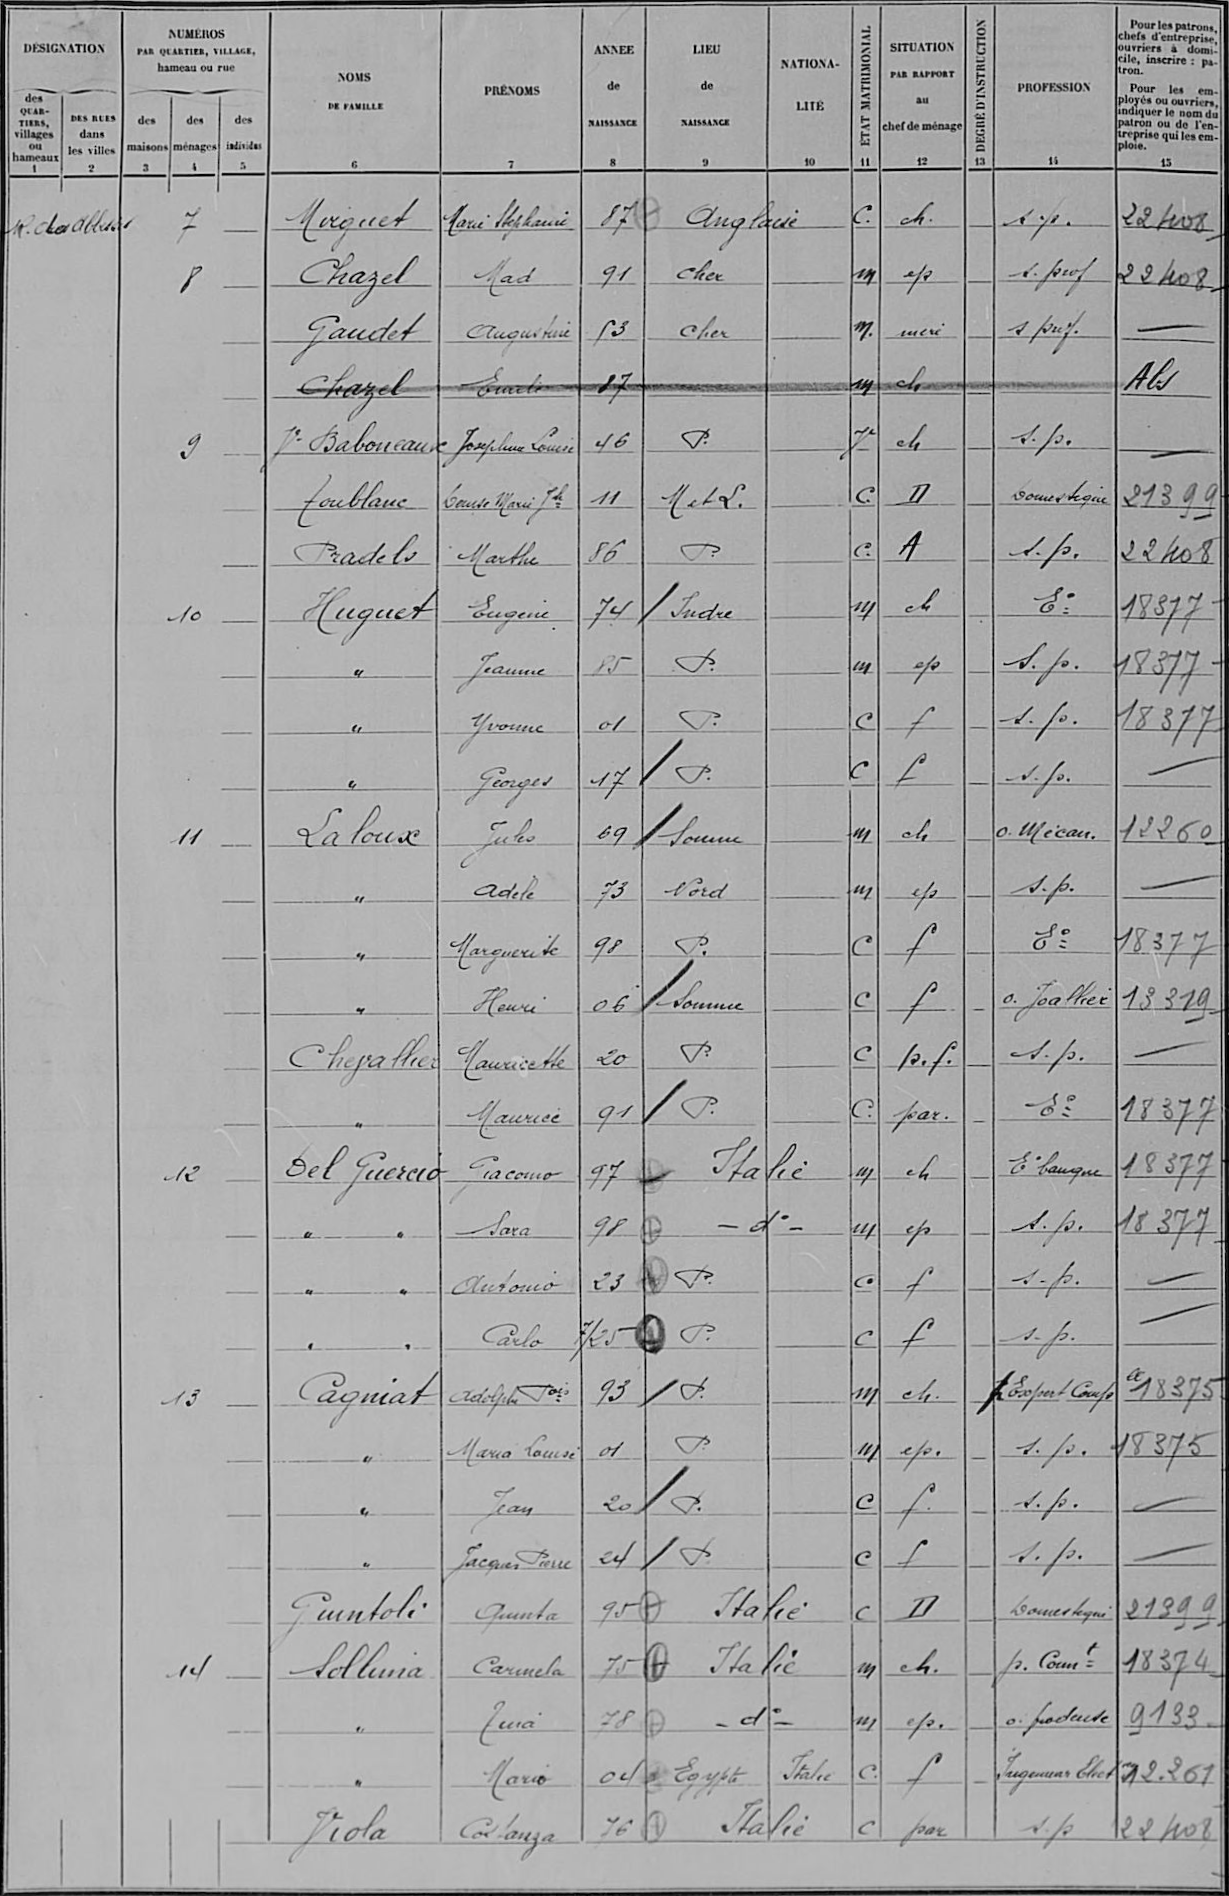Mirguet/Marie Stéphanie/87/Anglaise/¤/C /ch /¤/s p /22408
Chazel/Mad/91/cher/¤/m/ep/¤/s prof/22408
Gaudet/Augustine/53/cher/¤/m /mère/¤/s prof /¤
Chazel/Emile/87/¤/¤/m/ch/¤/¤/Abs
V Baboueaux/Joséphine Louise/46/P /¤/V/ch/¤/s p /¤
Toublanc/Louise Marie Jh/11/M et L /¤/C/D/¤/domestique/21399
Pradelo/Marthe/86/P /¤/C /A/¤/s p /22408
Huguet/Eugenie/74/Indre/¤/m/ch/¤/E°/18377
"/Jeanne/85/P /¤/m/ep /¤/s p /18377
"/Yvonne/01/P /¤/C/f/¤/s p /18377
"/Georges/17/P /¤/C/f/¤/s p /¤
Laloux/Jules/69/Somme/¤/m/ch/¤/o mécan /12260
"/Adèle/73/Nord/¤/m/ep/¤/s p /¤
¤/marguerite/98/P /¤/C/f/¤/E°/18377
¤/Henri/06/Somme/¤/C/f/¤/o joallier/13319
Chevallier/Mauricette/20/P/¤/C/p f /¤/s p /¤
¤/Maurice/91/P /¤/C /par /¤/E°/18377
Del Guercio/Giacomo/97/Italie/¤/m/ch/¤/E banque/18377
" "/Sara/98/d°/¤/m/ep/¤/s p /18377
" "/Antonio/23/P /¤/C/f/¤/s p /¤
" "/Carlo/7 25/P /¤/C/f/¤/s p /¤
Cagniat/Adolphe Pois/93/P /¤/m/ch /¤/Expert comp/18375
"/Maria Louise/01/P /¤/m/ep /¤/s p /18375
"/Jean/20/P /¤/C/f /¤/s p /¤
"/Jacques Pierre/24/P /¤/C/f/¤/s p /¤
Guintoli/Quinta/95/Italie/¤/C/D/¤/Domestique/21399
Sollunia/Carmela/75/Italie/¤/m/ch /¤/p Commt/18374
¤/Zuia/78/d°/¤/m/ep /¤/o brodeuse/9133
¤/Mario/04/Egypte/Italie/C/f/¤/Ingenieur chef/12 261
Viola/Costanza/76/Italie/¤/C/par/¤/s p /22408
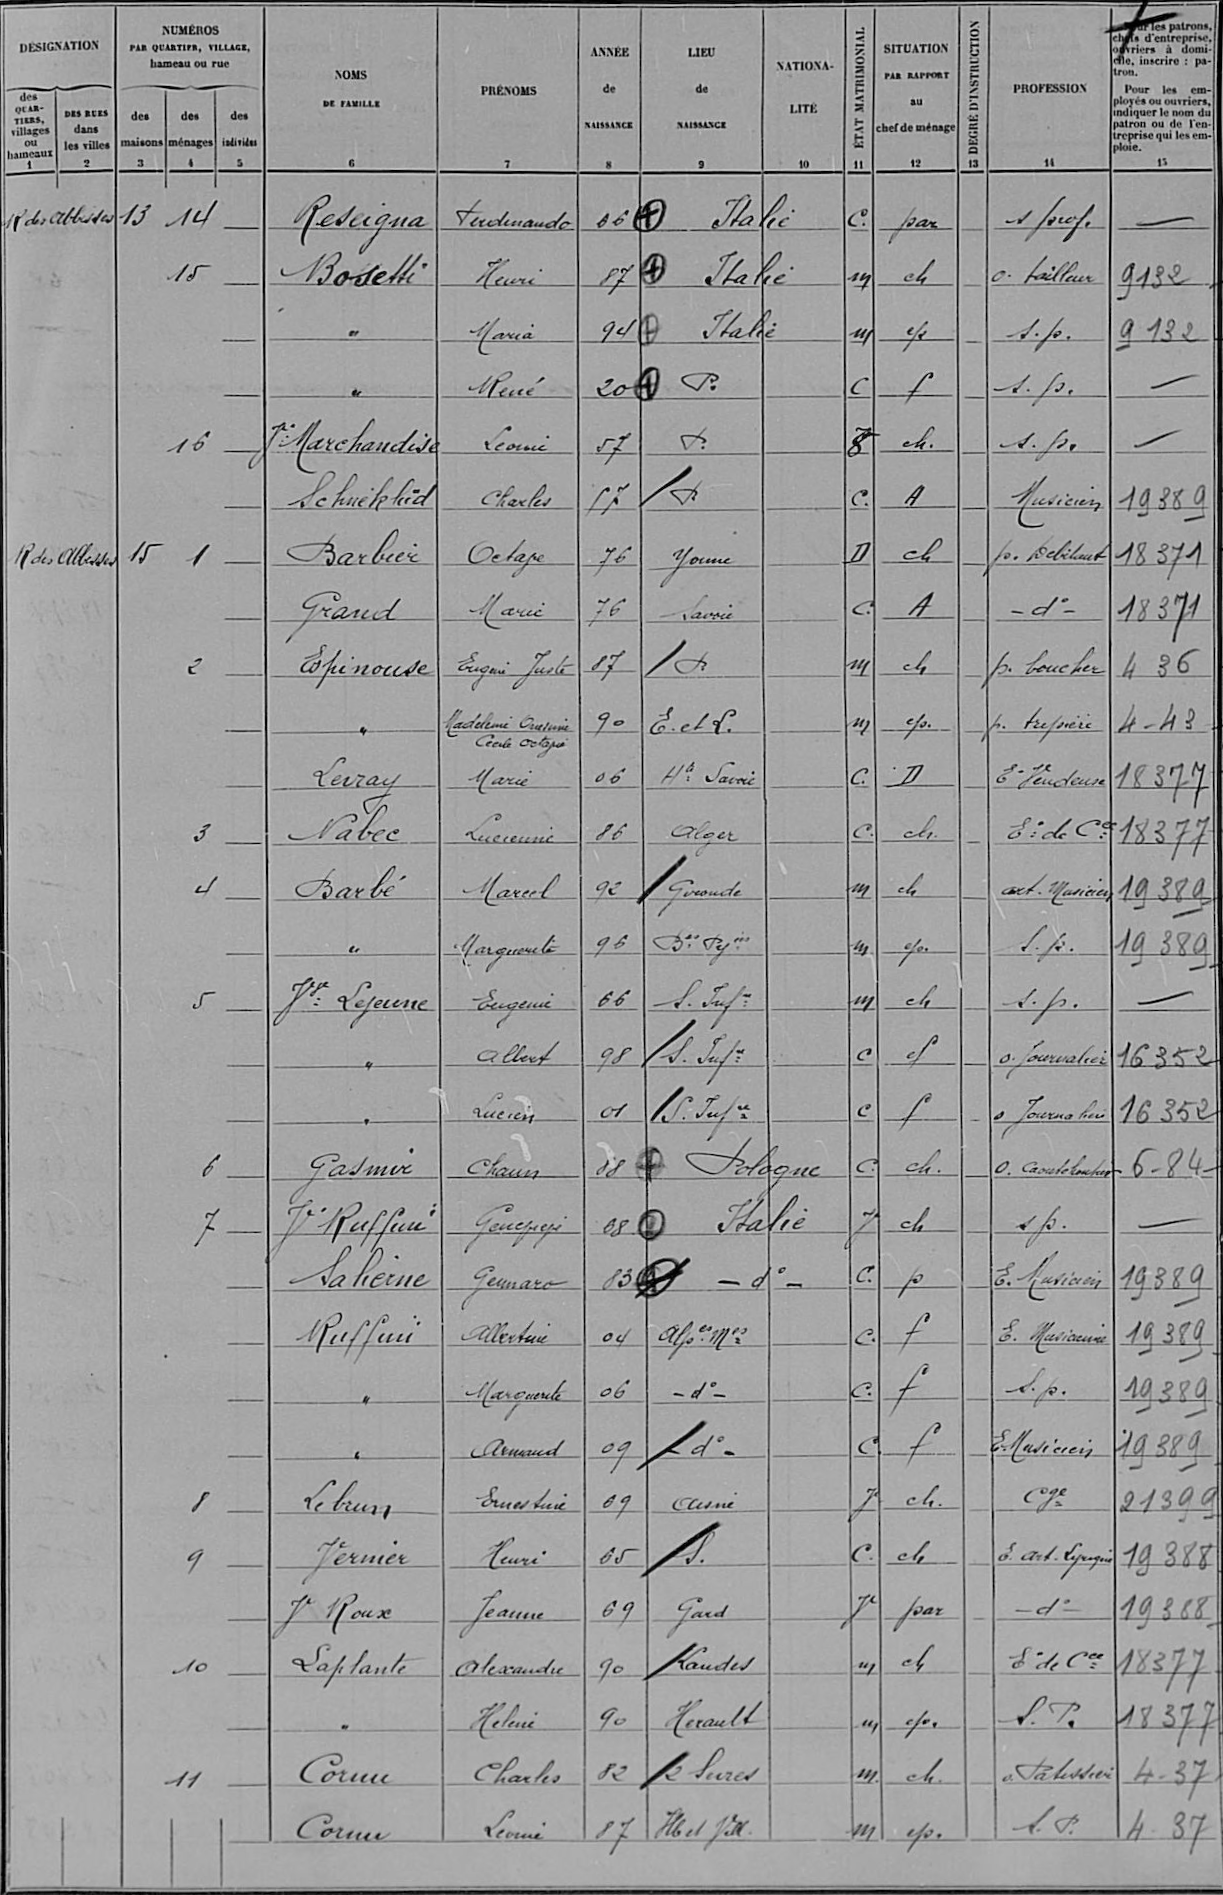Reseigna/Ferdinando/06/Italie/¤/C /par/¤/s prof /¤
Bosetti/Henri/87/Italie/¤/m/ch/¤/o tailleur/9132
¤/maria/94/Italie/¤/m/ep/¤/s p /9132
¤/René/20/P /¤/C/f/¤/s p /¤
V Marchandise/Leonie/57/P /¤/V/ch /¤/s p /¤
Schneklüd/Charles/57/P/¤/C /A/¤/Musicien/19389
Barbier/Octave/76/Yonne/¤/D/ch/¤/p Debitant/18371
Grand/Marie/76/Savoie/¤/C /A/¤/d°/18371
Espinouse/Eugénie Juste/87/P/¤/m/ch/¤/p boucher/436
¤/Madeleine Oresine !Cecile Octavie/90/E et L /¤/m/ep /¤/p fripière/4 43
Levray/Marie/06/Hte Savoie/¤/C /D/¤/E Vendeuse/18377
Nabec/Lucienne/86/Alger/¤/C /ch /¤/E de Cce/18377
Barbé/Marcel/92/Gironde/¤/m/ch/¤/art Musicien/19389
¤/Marguerite/96/Bes Pées/¤/m/ep /¤/s p /19389
Veuve Lejeune/Eugénie/66/S Infre/¤/m/ch/¤/s p /¤
¤/albert/98/S Infre/¤/c/f/¤/o journalier/16352
¤/Lucien/01/S Infre/¤/c/f/¤/o journalier/16352
Gasmir/Chaun/88/Pologne/¤/C /ch /¤/O Caoutchouteur/6 84
V Ruffini/Genepiepi/68/Italie/¤/V/ch/¤/sp /¤
Salierne/Gennaro/83/d°/¤/C /p/¤/E Musicien/19389
Ruffini/Albertine/04/Alpes Mes/¤/C/f/¤/E Musicienne/19389
¤/Marguerite/06/d°/¤/C /f/¤/s p /19389
¤/Armand/09/d°/¤/C /f/¤/E Musicien/19389
Lebrun/Ernestine/09/Aisne/¤/V/ch /¤/Cge/21399
Vernier/Henri/05/S/¤/C /ch/¤/E art Le progrès/19388
V Roux/Jeanne/69/Gard/¤/V/par/¤/d°/19388
Laplante/Alexandre/90/Landes/¤/m/ch/¤/E de Cce/18377
¤/Helene/90/Herault/¤/m/ep /¤/S P /18377
Cornu/Charles/82/2 Sevres/¤/m /ch /¤/o Patissier/4 37
Cornu/Leonie/87/Ille et Vill/¤/m/ep /¤/s p /4 37